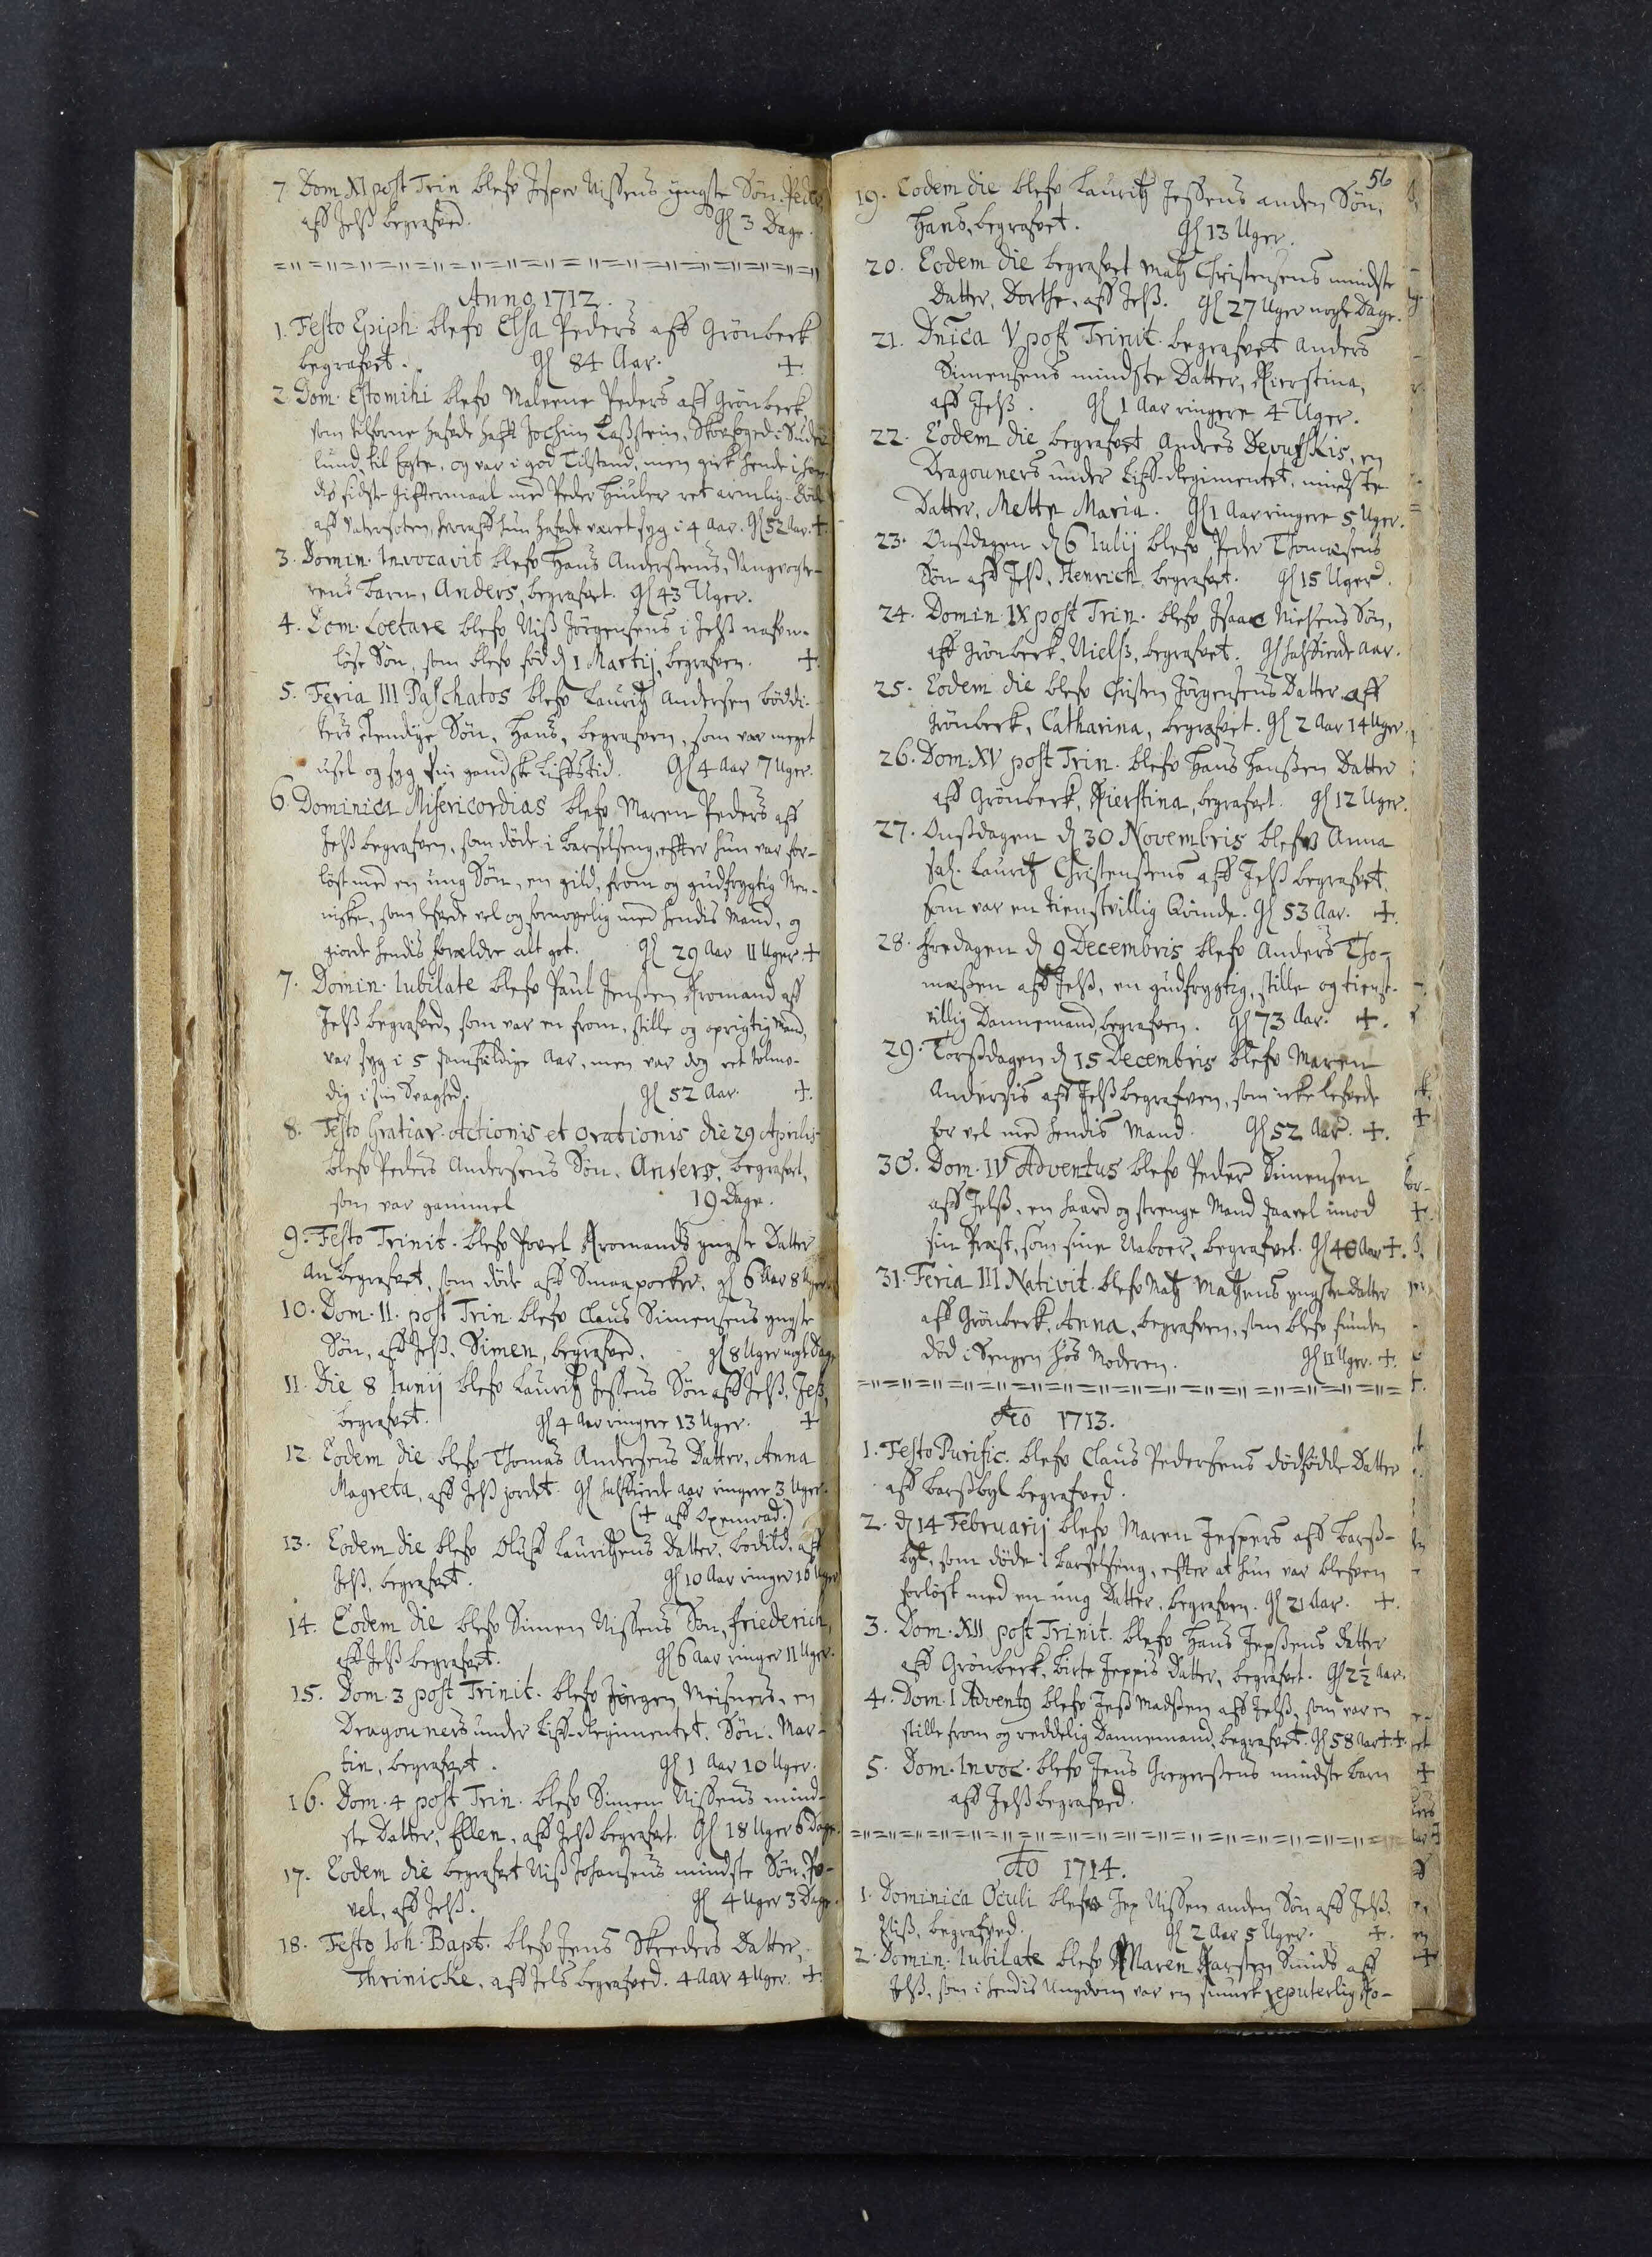7. Dom XI post Trin blefv Jesper Nissens yngste Søn, Peder
aff Jelss begrafved.
Gl 3 Dage
Anno 1712
1. Festo Epiph blefv Elsa Peders aff Grønbeck
begrafvet.
Gl 84 Aar.
2. Dom. Esto mihi blefv Maleene Peders aff Grønbeck
som tilforne hafvde hafft Jochim Lasstein, Skovfoged i Suder
lund, til Egte, og var i god Tilstand, men gick hende i hen
dis sidste giftermaal med Peder Hiuler ret armlig##. Døde
aff Vatersoten, hvoraff hun hafvde været syg i 4 Aar. Gl 52 Aar.
3. Domin. Invocavit blefv Hans Anderssens, Vangvogte¬
rens barn, Anders, begrafvet. Gl 43 Uger.
4. Dom. Loetare blefv Niss Jørgensens i Jelss nafvn¬
løse Søn, som blefv fød 1 Martij, begrafven.
5. Feria III Paschatos blefv Lauritz Andersen Bøddi¬
kers elendige Søn, Hans, begrafven, som var meget
usel og syg sin gandske Liffstid. Gl 4 Aar 7 Uger.
6. Dominica Misericordias blefv Maren Peders aff
Jelss begrafven, som døde i Barselseng effter hun var for¬
løst med en ung Søn, en gild, from og gudfrygtig Men¬
niske, som lefede vel og fornoyelig med hendis Mand, og
giorde hendis Forældre alt got. Gl 29 Aar 11 Uger
7. Domin. Iubilate blefv Paul Jenssen Kromand aff
Jelss begrafved, som var en from, stille og oprigtig Mand,
var syg i 5 samfuldige Aar, men var dog ret tolmo¬
dig i sin Svaghed.
Gl 52 Aar
8. Festo Gratiar. Actionis et orationis die 29 Aprilis
blefv Peder Andersens Søn, Anders, begrafvet,
som var gammel
19 Dage.
9. Festo Trinit. blefv Povel Kromands yngste Datter
An begrafvet, som døde aff Smaapocker. gl 6 Aar 8 Uger
10. Dom. II post Trin blefv Claus Simensens yngste
Søn, aff Jelss, Simen, begrafved. gl 8 Uger nogle Dage
11. Die 8 Iunij blefv Lauritz Jessens Søn aff Jelss, Jess,
begrafvet.
gl 4 Aar ringere 13 Uger.
12. Eodem die blefv Thomas Andersens Datter, Anna
Magreta, aff Jelss jordet. Gl halffierde Aar ringere 3 Uger.
aff Oxenvad.)
13. Eodem die blefv Oluff Lauritzens Datter, Bodild aff
Jelss, begrafvet.
Gl 10 Aar ringer 10 Uger
14. Eodem die blefv Simen Nissens Søn, Friederich,
aff Jelss, begrafvet.
Gl 6 Aar ringer 11 Uger
15. Dom 3 post Trinit. blefv Jørgen Meisners, en
Dragouners under Liff-Regimentet, Søn, Mar
tin, begrafvet.
Gl 1 Aar 10 Uger.
16. Dom. 4 post Trin. blefv Simen Nissens mind¬
ste Datter, Ellen, aff Jelss begrafvet. Gl 18 Uger 6 Dage
17. Eodem die begrafvet Nis Johansens mindste Søn, Po¬
vel, aff Jelss.
Gl 4 Uger 3 Dage
18. Festo Ioh. Bapt. blefv Jens Skreders Datter,
Thrinicke, aff Jels begrafved. 4 Aar 4 Uger.
56
19. Eodem die blefv Lauritz Jessens anden Søn,
Hans, begrafvet. Gl 13 Uger.
20. Eodem die begrafvet Matz Christensens mindste
Datter, Dorthe, aff Jelss. Gl 27 Uger nogle Dage.
21. Dnica V post Trinit. begrafvet Anders
Simensens mindste Datter, Kierstina,
aff Jelss. Gl 1 Aar ringere 4 Uger.
22. Eodem die begrafvet Anders Devuthkis, en
Dragouners under Liff-Regimentet, mindste
Datter, Mette Maria. Gl 1 Aar ringere 5 Uger.
23. Onssdagen d 6 Iulij blefv Peder Thomasens
Søn aff Jelss, Henrich, begrafvet. Gl 15 Uger
24. Domin. IX post Trin. blefv Isaac Nielsens Søn,
aff Grønbeck, Nielss, begrafvet. Gl halffierde Aar.
25. Eodem die blefv Christen Jørgensens Datter aff
Grønbeck, Catharina, begrafvet. Gl 2 Aar 14 Uger.
26. Dom. XV post Trin. blefv Hans Hanssen Datter
aff Grønbeck, Kierstina, begrafvet. Gl 12 Uger
27. Onssdagen d 30 Novembris blefv Anna
sal. Lauritz Christenssens aff Jelss begrafvet
som var en tienstvillig Qvinde. Gl 53 Aar.
28. Fredagen d 9 Decembris blefv Anders Tho¬
masen aff Jelss, en gudfrygtig, stille og tienst¬
villig Dannemand, begrafven. Gl 73 Aar.
29. Torssdagen d 15 Decembris blefv Maren
Andersis aff Jelss begrafven, som icke lefvede
for vel med hendis Mand. Gl 52 Aar
30. Dom. IV Adventus blefv Peder Simensen
aff Jelss, en haard og strenge Mand saavel imod
sin Præst, som sine Naboer, begrafvet. Gl 40 Aar
31. Feria III Nativit. blefv Matz Matzens yngste Datter
aff Grønbeck, Anna, begrafven, som blefv funden
død i sengen hos Moderen
Gl 11 Uger.
Ao 1713.
1. Festo Purific. blefv Claus Pedersens dødfødde Datter
aff Barsbyl begrafved
2. d 14 Februarij blefv Maren Jespers aff Barss
byl, som døde i barselseng, efter at hun var blefven
forløst med en ung Datter, begrafven. Gl 21 Aar.
3. Dom. XII post Trinit. blefv Hans Jepssens Datter
aff Grønbeck, Birte Jeppis Datter, begrafvet. Gl 2½ Aar.
4. Dom. I Adventq blefv Jess Madssen aff Jelss, som var en
stille from og reddelig Dannemand, begrafvet. Gl 58 Aar
5. Dom. Invoc. blefv Jens Gregerssens mindste barn
aff Jelss begrafved.
Ao 1714.
1. Dominica Oculi blefv Jep Nissen anden Søn aff Jelss,
Niss, begrafved
Gl 2 Aar 5 Uger.
2. Domin. Iubilate blefv Maren Karsten Smids aff
Jelss, som i hendis Ungdom var en smuck reputerlig Ko¬